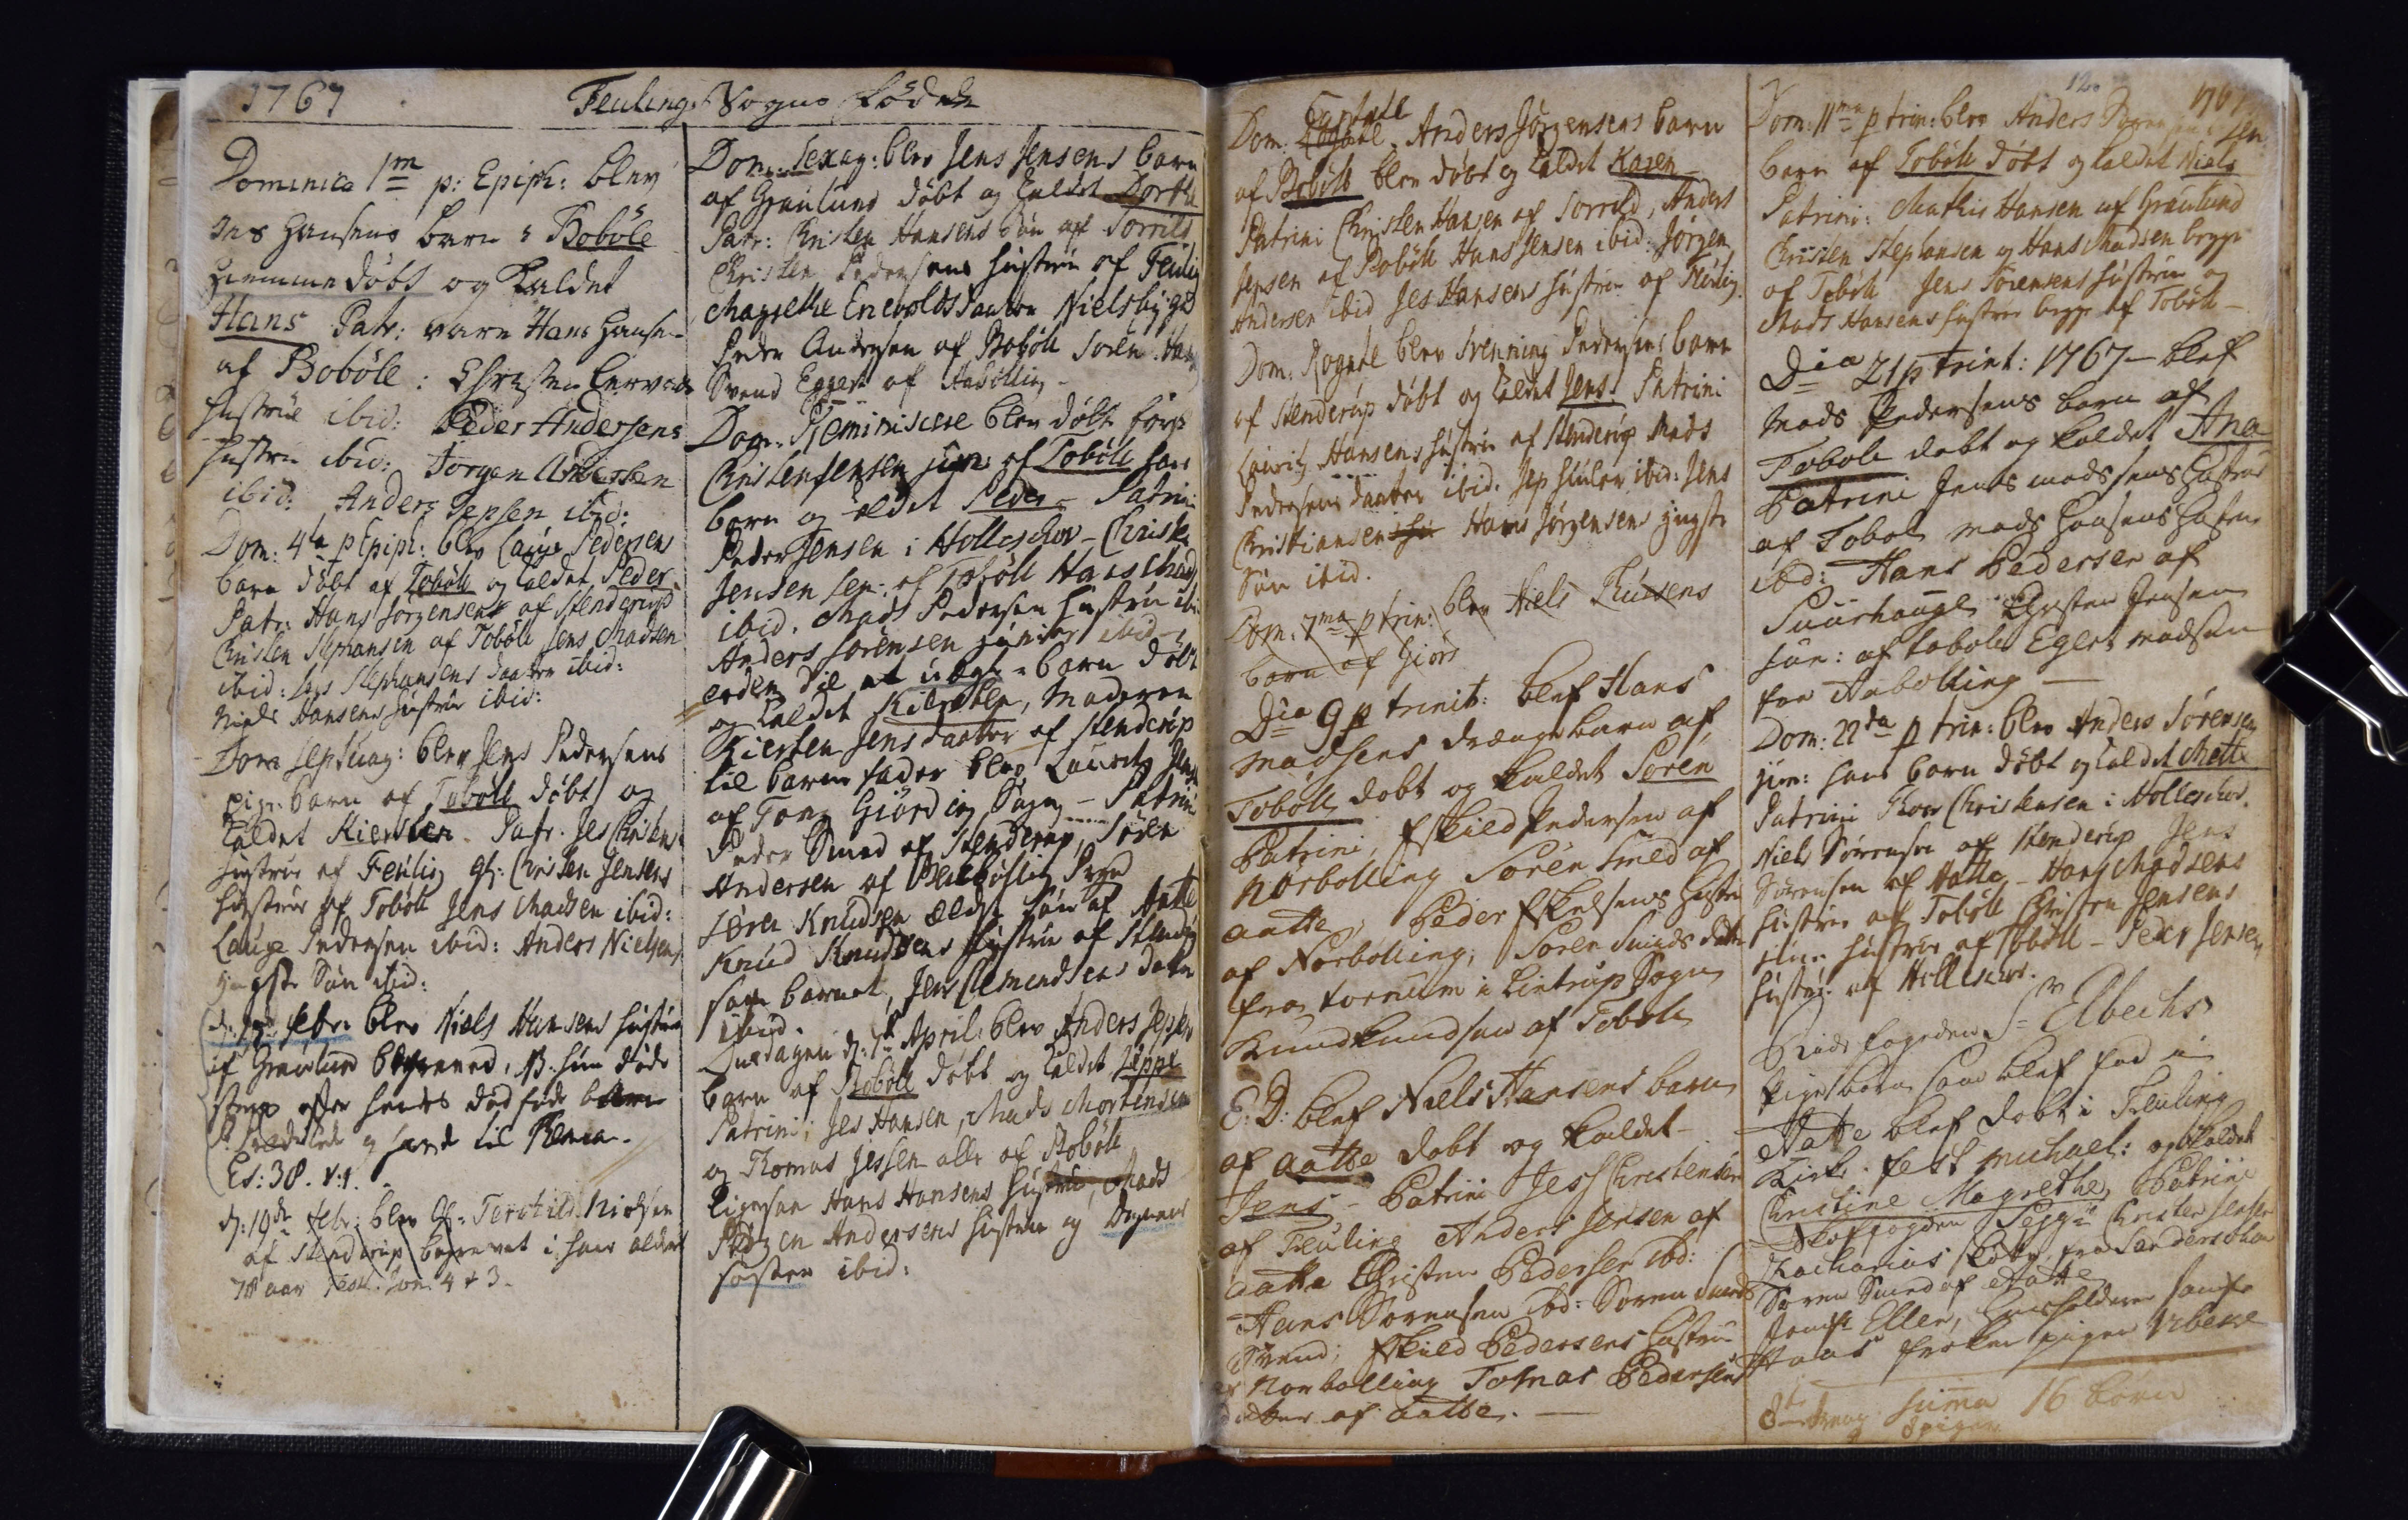1767
Feuling Sogns Fødde
Dominica 1ma p: Epiph: blev
Jes Hansens barn i Bobøll
Hiemmedøbt og kaldet
Hans Patr; vare Hans Hansen
af Bobøll: Christen Lervads
Hustrue ibid: Peder Andersens
Hustru ibid: Jørgen Andersen
ibid: Anders Jepsen ibid:
Dom: 4ta p Epiph: blev Lauge Pedersens
barn døbt af Tobøll og kaldet Peder.
Patr: Hans Jørgensen af Stenderup
Christen Stephansen af Tobøll Jens Madsen
ibid: Lars Stephansens Daatter ibid:
Niels Hansens Hustrue ibid:
Dom Septuag: blev Jens Pedersens
pige barn af Tobøll døbt og
kaldet Kiersten. Patr. Jes Christens
Hustrue af Feuling gl: Christen Jensens
Hustrue af Tobøll Jens Madsen ibid:
Lauge Pedersen ibid: Anders Nielsens
H##ste Søn ibid
d: ##de Febr: blev Niels Hansens Hustrue
af Graulund begraved, NB: han døde
strax efter hendes dødfød barn
Prædike og havde til K##
Et: 38. v: 1
d: 19de Febr: blev Sl: Terchild Nielsen
af Stenderup begravet i hans alders
78 aar Fest. Tom 4 + 3.
Dom: Sexag: blev Jens Jensens barn
af Graulund døbt og kaldet Dorthe.
Pat: Christen Hansens Søn af Sorrild
Christen Pedersens Hustrue af Feuling
Magrete Enevoldsdaatter Nielsby grd
Peder Andersen af Bobøll Søren Han##
Svend Eggert af Aabølling
Dom Reminiscere blev døbt f##
Christensen jun af Tobøll hans
barn og kdet Peder Patrini
Peder Jensen i Holleschov Christen
Jensen sen af Tobøll Hans Mads
ibid: Madz Pedersens Hustru ibid
Anders Sørensen junior ibid.
eodem Die et uægte = barn døbt
og kaldet Kiersten, Moderen
Kiersten Jens daatter af Stenderup
til barnefader blev Lauritz Jen##
af Tong Giording Sogn - Patrini
Peder Smed af Stenderup, Søren
Andersen af Bechbølling Sogn
Søren Knudsen ældst Søn af Aatte
Knud Knudsens Hustrue af Stenderup
som barnet, Jens Clemendsens Datter
Jbid.
Onsdagen d: 7de April: blev Anders Jepsens
barn af Bobøll døbt og kaldet Jeppe
Patrini; Jes Hansen, Mads Mortensen
og Thomas Jessen alle af Bobøll
Ligesaa Hans Hansens Hustru Mads
P## Andersens Hustrue og Degen
søster ibid:
Cantate
Dom: ## Anders Jørgensens barn
af Bobøll blev døbt og kaldet Karen
Patrini Christen Hansen af Sorrild, Anders
jepsen i Bobøll Hans Jensen ibid: Jørgen
Andersen ibid Jes Hansens Hustrue af Feulingi
Dom: Rogate blev Svenning Pedersens barn
af Stenderup døbt og kaldet Jens Patrini
Lauritz Hansens Hustrue af Stenderup, Mads
Pedersens datter ibid. Jep hiuler ibid: Jens
Christiansen her Hans Jørgensens yngste
Søn ibid.
Dom: 7ma p trin: blev Niels Thuesens
barn af Giors
Dca 9 p trinit: Blef Hans
Madsens drenge barn af
Tobøll døbt og kaldet Søren
Patrini Eskild Pedersen af
Nørbølling Søren Smed af
Aatte, Peder Eskelsens Hustr
af Nørbølling, Søren Smids datter
fra forum i Lintrup Sogn
Knud Knudsen af Tobøll
E: D: blef Niels Hansens barn
af Aatte døbt og kaldet
Jens, Patrini Jes Christensen
af Feuling Anders Jensen af
Aatte Christen Pedersen ibd:
Hans Sørensen ibd: Søren Smeds
Svend; Eskild Pedersens Hustrue
af Norbolling, Tomas Pedersens
Datter af Aatte.
12
1767
Dom: 11ma p: Trin: blev Anders Sørensens sen
Barn af Tobøll døbt og kaldet Niels
Patrini: Mathis Hansen af Graulund
Christen Stephansen og Hans Madsen begge
af Tobøll Jens Sørensens Hustrue og
Mads Hansens Hustrue begge af Tobøll
Dca 21 p Trinit: 1767 -  blef
Mads Pedersens barn af
Toboll døbt og kaldet Ana
Patrini Jens Madssens Hustrue
af Tobol, Mads Hansens Hustrue
ibd: Hans Pedersen af
Suurhauge Christen Jensen
jun: af toboll Egers Madsen
fra Aabolling
Dom: 22da p Trin: blev Anders Sørensen
jun: hans barn døbt og kaldet Mette
Patrini Hans Christensen i Holleschov
Niels Sørensen af Stenderup Jens
Sørensen af Aatte -  Hans Madsens
Hustru af Tobøll Christen Jensens
juns Hustrue af Tobøll - Peder Jensens
Hustru af Holleschov.
Ridefogeden Sr Elbechs
Pigebarn, som blef fød i
Aatte blef døbt i Feuling
Kirke fest Michael: og kaldet
Christine Magrethe, Patrini
Skoffogden Sejgr Christen Jensen
Zacharias Skøtte, fra Sandersckov
Søren Smed af Aatte
Jomfr Ellen, Husholderen Jomfr
Haas froken pigen Abeke
8te drenge summa 16 børn
## Piger
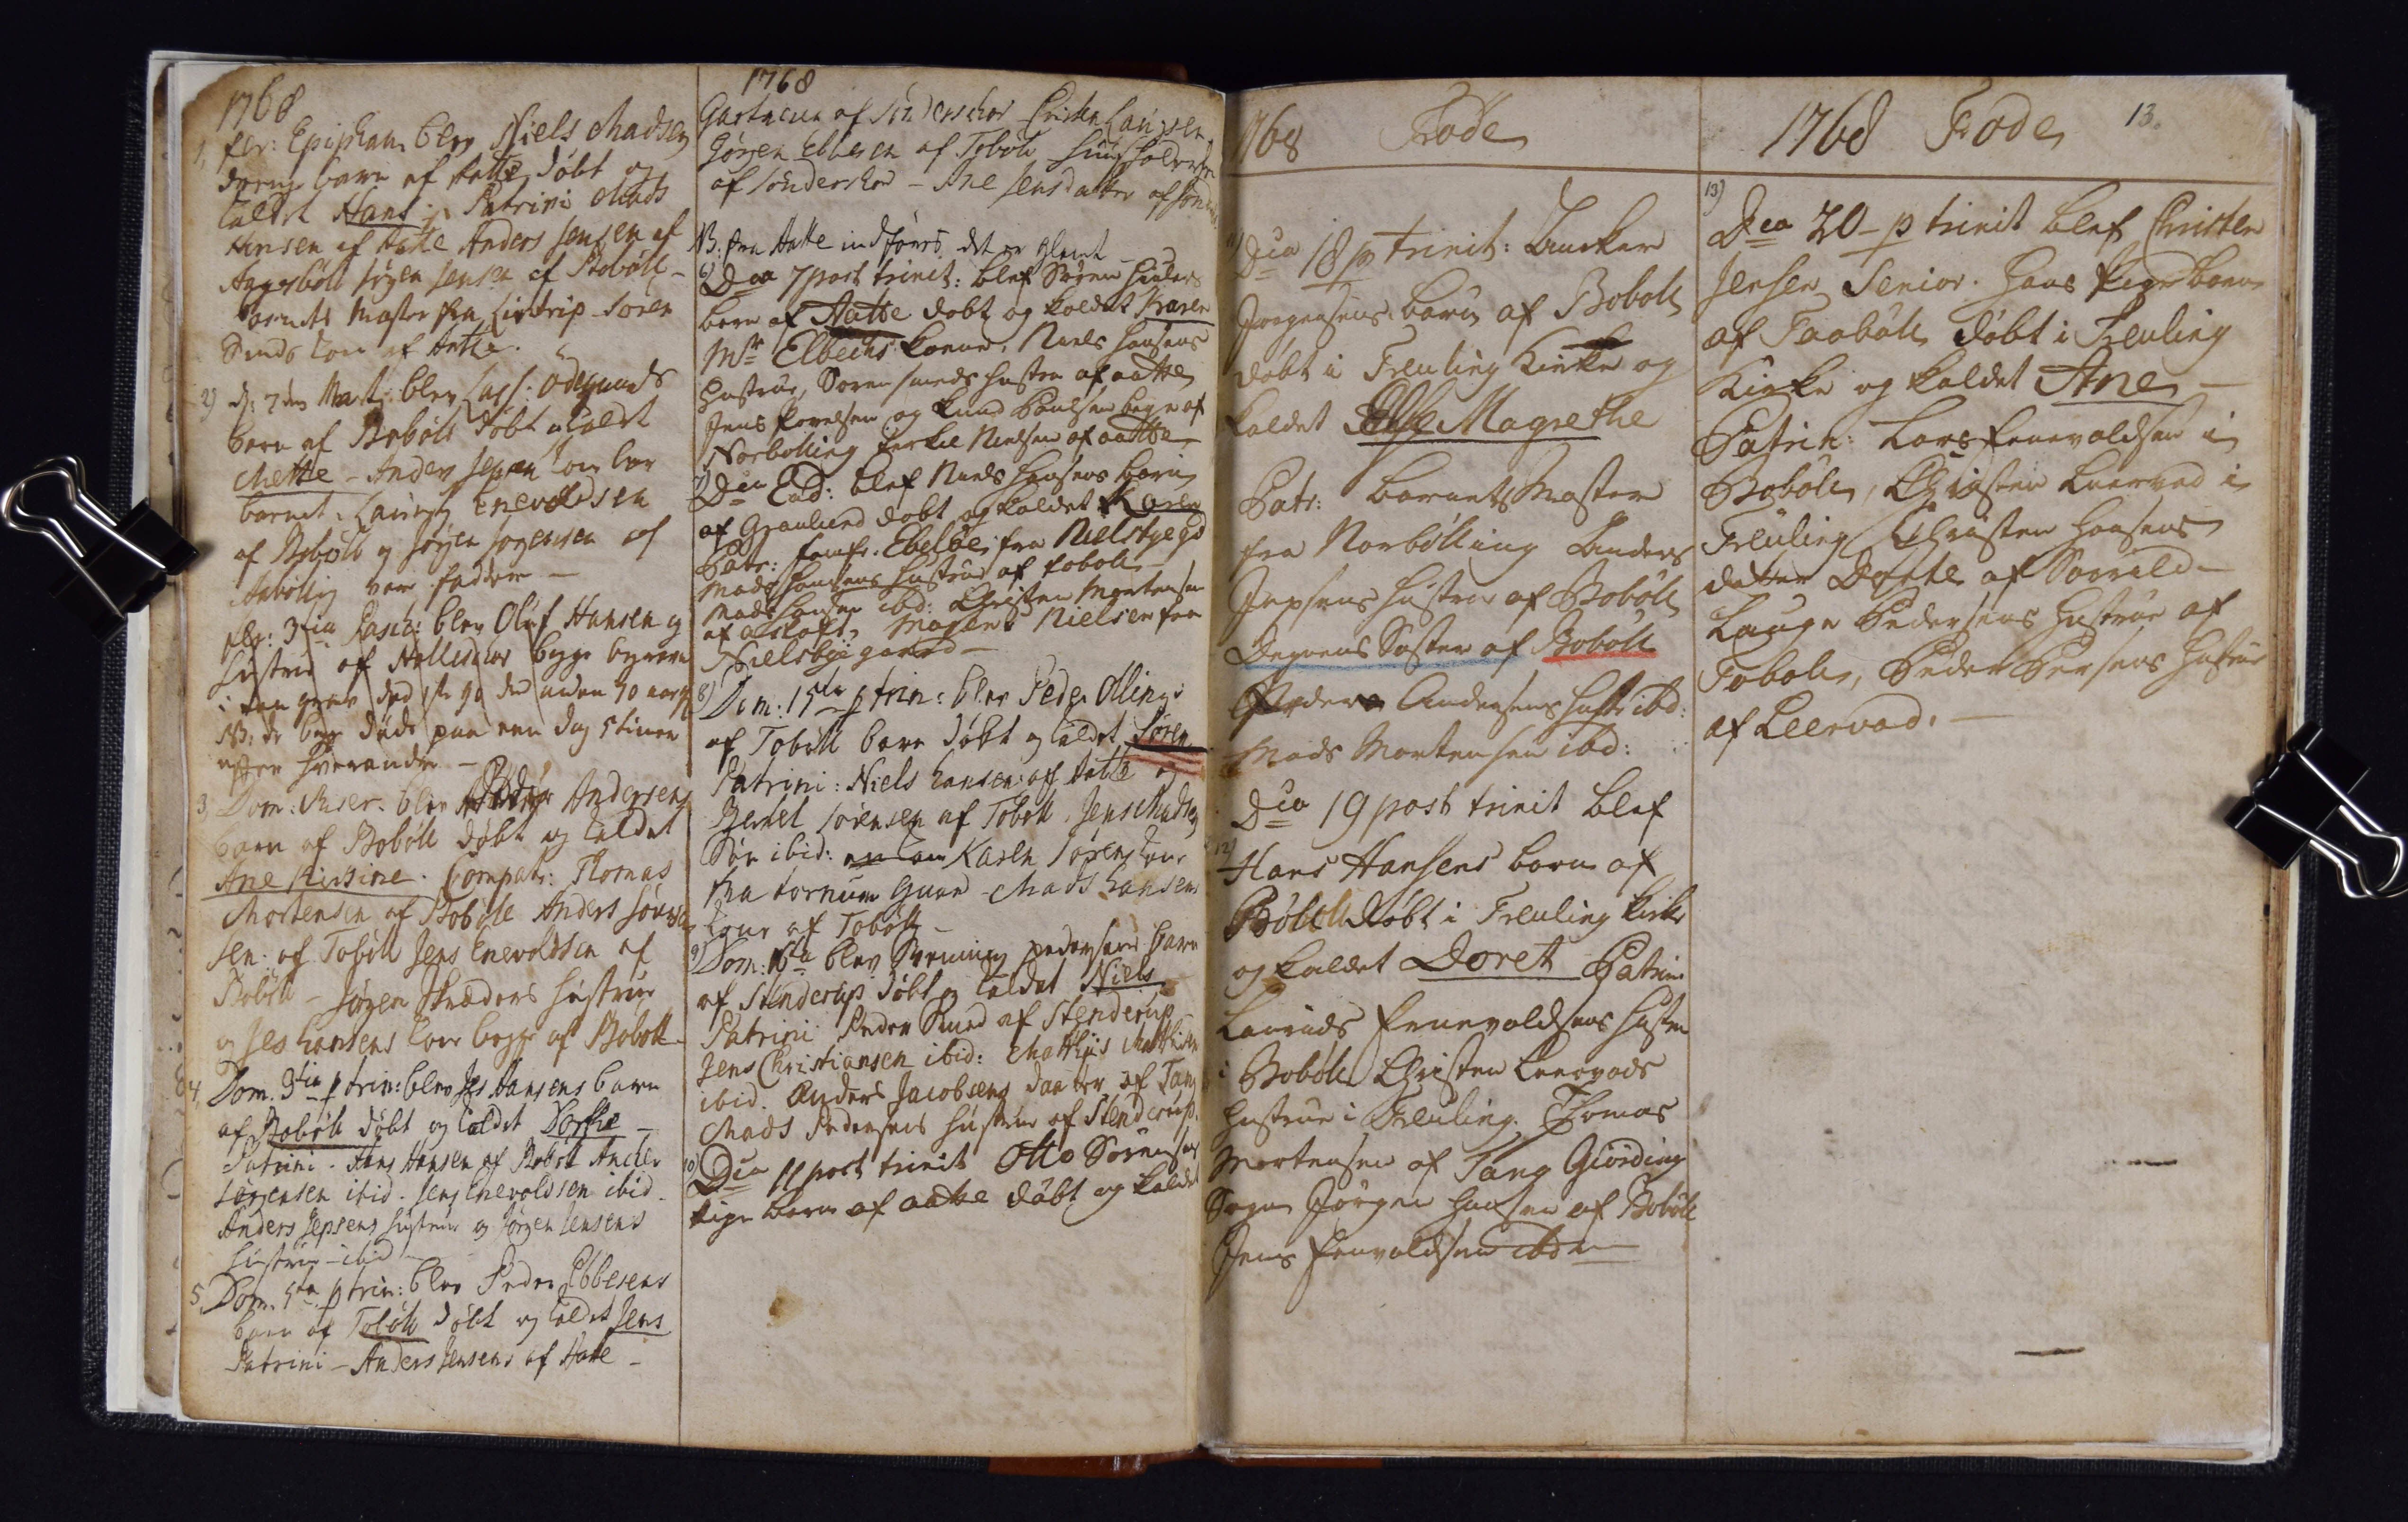1768
1, Fer: Epiphan: blev Niels Madsens
drenge barn af Aatte døbt og
kaldet Hans; Patrini Mads
Hansen af Aatte Anders Jensen af
Aggesbøll Jørgen Jensen af Bobøll -
barnets Moster fra Linstrup Soren
Smeds kone af Aatte
2) d: 7de Martj blev Lass Ødegaards
barn af Bobøll døbt og kaldet
Mette - Anders Jepsen kone bar
barnet. Lauritz Envoldsen
af Bobøll og Jørgen Jørgensen af
Aabølling som faddere -
Fer: 3tia Pasch: blev Oluf Hansen og
Hustru af Holleschov begge begraven
i ## ## dend 1st 90 den anden 70 aar gl 
NB: de begge døde paa een dag 5 timer
efter hverandre
3, Dom: Miser: blev Peder Andersens
barn af Bobøll døbt og kaldet
Anne Kirstine Compatr: Thomas
Mortensen af Bobøll Anders Jørgen
sen af Tobøll Jens Envoldsen af
Bobøll - Jørgen Skræders hustrue
og Jes Hansens Kone begge af Bobøll
4, Dom: 9tia p Trin: blev Jens Hansens Barn
af Bobøll døbt og kaldet Dorthe
Patrini. Hans Hansen af Bobøll Ancher
Jørgensen ibid Jens Enevoldsen ibid.
Anders Jensens Hustrue og Jørgen Jensens
Hustruer - ibid
5, Dom: 5ta p Trin: blev Peder Ebbesens
barn af Tobøll døbt og kaldet Jens
Patrini - Anders Jensens af Aatte
1768
Gart## af Sonderschov - Christen Laugesen
Jørgen Ebbesen af Tobøll Huusholdersken
af Søndewrskov - Ane Jensdatter af ##
NB: fra Aatte indføres det er glemt
6) Dca 7 post Trinit: blef Søren Hiulers
barn af Aatte døbt og kaldet Karen
Mr Elbechs Koene Niels Hansens
Hustrue, Søren smeds Hustru af Aatte
Jens Povelsen og Knud Poulsen begge af
Norbølling tirkel Nielsen af aatte
7) Dca Eod: bleff Niels Hansens barn
af Graulund dobt og kaldet Karen
Patr: Jomfr: Ebeløe fra Nielsbye gd
Madz Hansens Hustrue af Toboll
Mads Hansen ibid: Christen Mortensen
af astoft, Maren Nielsen fra
Nielsbye gaard
8) Dom: 15ta p Trin: blev Peder Ollings
af Tobøll barn døbt og kaldet Søren
Patrini: Niels Hansen af Aatte og
Bertel Sørensen af Toboll, Jens Madsens
Søn ibid: en Kone Karen Sørens kone
fra fornum Gaard Mads Hansens
Kone af Tobøll
9) Dom: 16ta blev Svenning Pedersens barn
af Stenderup døbt og kaldet Niels
Patrini Peder Smed af Stenderup
Jens Christiansen ibid: Mathiis Mattisen
ibid: Anders Jacobsens Daatter af Tang
Mads Pedersens Hustrue af Stenderup.
Dca 11 post trinit Otto Sørensens
Pige Barn af Aatte døbt og kaldet
Føde
1768
11) Dca 18 p Trinit: Ancker
Jørgensens barn af Boboll.
døbt i Feuling Kirke og
kaldet Else Magrethe
Patr: Barnets Moster
fra Norbølling Anders
Jepsens Hustru af Bobøll
Degnens Soster af Bobøl
Peder Andersens Hustr ibd:
Mads Mortensen ibd:
Dca 19 post Trinit. Blef
12
Hans Hansens barn af
Bøll ## døbt i Feuling Kirke
og kaldet Doret Patrin
Laurids Enevoldsens Hustru
i Bobøll, Christen Leervads
Hustrue i Feuling. Thomas
Mortensen af Tang Giording
Sogn Jørgen Hansen af Bobøll
Jens Enevoldsen ibd##
13.
1768 Fode
13)
Dca 20_ p Trinit blef Christen
Jensen Senior. Hans Pige Barn.
af Taabølle døbt i Feuling
Kirke og kaldet Ane
Patrin: Lars Eenevoldsen i
Bobøll, Christen Leervad i
Feuing, Christen Larsens
datter Dorte af Sorrild -
Lauge Pedersens Hustrue af
Toboll. Peder Persens Hustru
af Leervad.